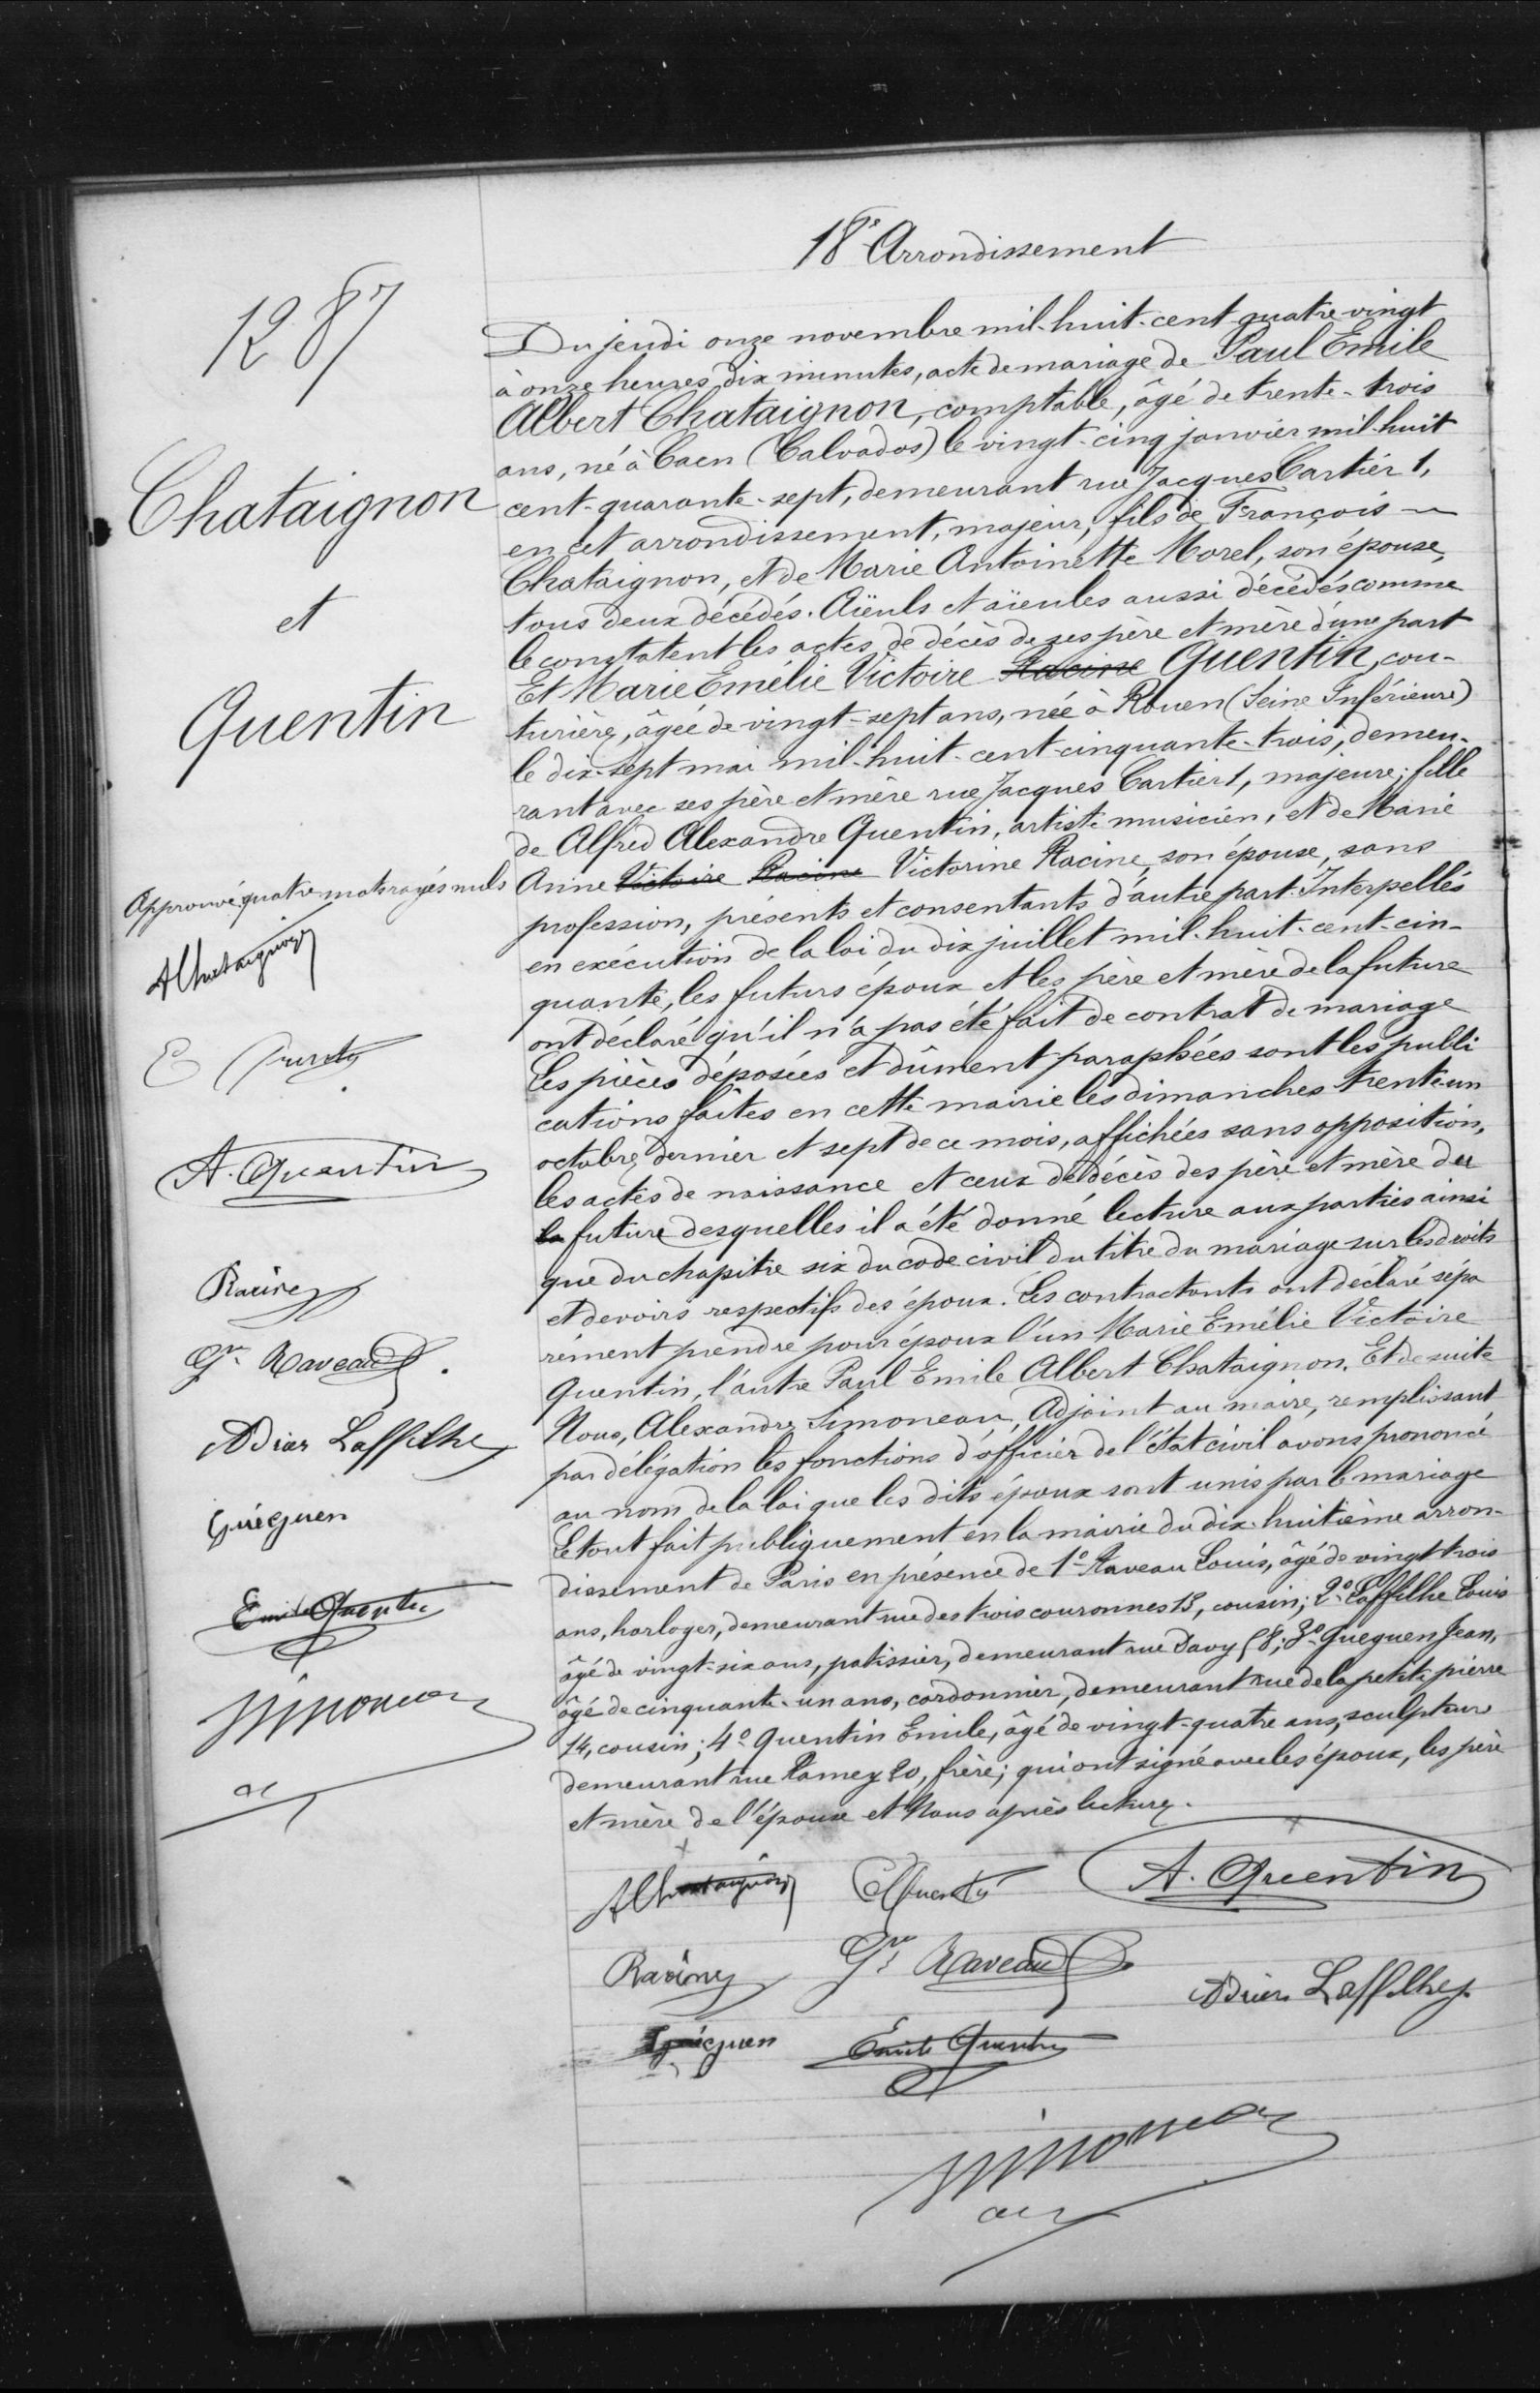1287
Chataignon
et
Quentin
18° Arrondissement
Du jeudi onze novembre mil-huit-cent-qautre vingt
à onze heures dix minutes, acte de mariage de Paul Emile
Albert Chataignon, comptable, âgé de trente-trois
ans, né à Caen (Calvados) le vingt-cinq janvier mil-huit
cent-quarante-sept, demeurant rue Jacques Cartier 1,
en cette arrondissement, majeur, fils de François -
Chataignon, et de Marie Antoinette Morel, son épouse,
tous deux décédés. Aïeuls et aïeules aussi décédés, comme
le constatent les actes de décès de ses père et mère d'une part
Et Marie Emilie Victoire Adeline Quentin, cou-
turière, âgé de de vingt-sept ans, née à Rouen (Seine Inférieure)
le dix-sept mai mil-huit-cent-cinquante-trois, demeu-
rant vec ses père et mère rue Jacques Cartier 1, majeure, fille
de Alfred Alexandre Quentin, artiste musicien, et de Marie
Anne Victoire Racine Victorine Racine, son épouse, sans
profession, présents et consentant d'autre part. Interpellés
en exécution de la loi du dix juillet mil-huit-cent-cin-
quante, les futurs époux et les père et mère de la future
ont déclaré qu'il n'a pas été fait de contrat de mariage
Les pièces déposées et dûment paraphées sont les publi
cations faites en cette mairie les dimanches trente-un
octobre dernier et sept de ce mois, affichées sans opposition,
les actes de naissance et ceux de décè des père et mère du
la future desquelles il a été donné lecture aux parties ainsi
que du chapitre six du code civildu titre du mariage sur les droits
et devoirs respectifs des époux. Les contractants ont déclaré sépa
rément prendre pour époux l'un Marie Emilie Victoire
Quentin, l'autre Paul Emile Albert Chataignon, Et de suite
Nous, Alexandre Simoneau, Adjoint au maire, remplissant
par délégation les fonctions d'officier de l'état civil avons prononcé
au nom de la loi que les dits époux sont unis par le mariage
Le tout fait publiquement en la mairie du dix-huitième arron-
dissement de Paris en présence de 1° Raveau Louis, âgé de vingt-trois
ans, horloger, demeurant rue des trois couronnes, 13, cousin; 2° Laffilhe Louis
âgé de vingt-six ans, patissier, demeurant ru Savy 58; 3° Gueguen Jean,
âgé de cinquante-un ans, cordonnier, demeurant rue de la petite pierre
14, cousin; 4° Quentin Emile, âgé de vingt-quatre ans, sculpteur
demeurant rue Lamey 20, frère; qui ont signé avec les époux, les père
et mère de l'époux et Nous après lecture.
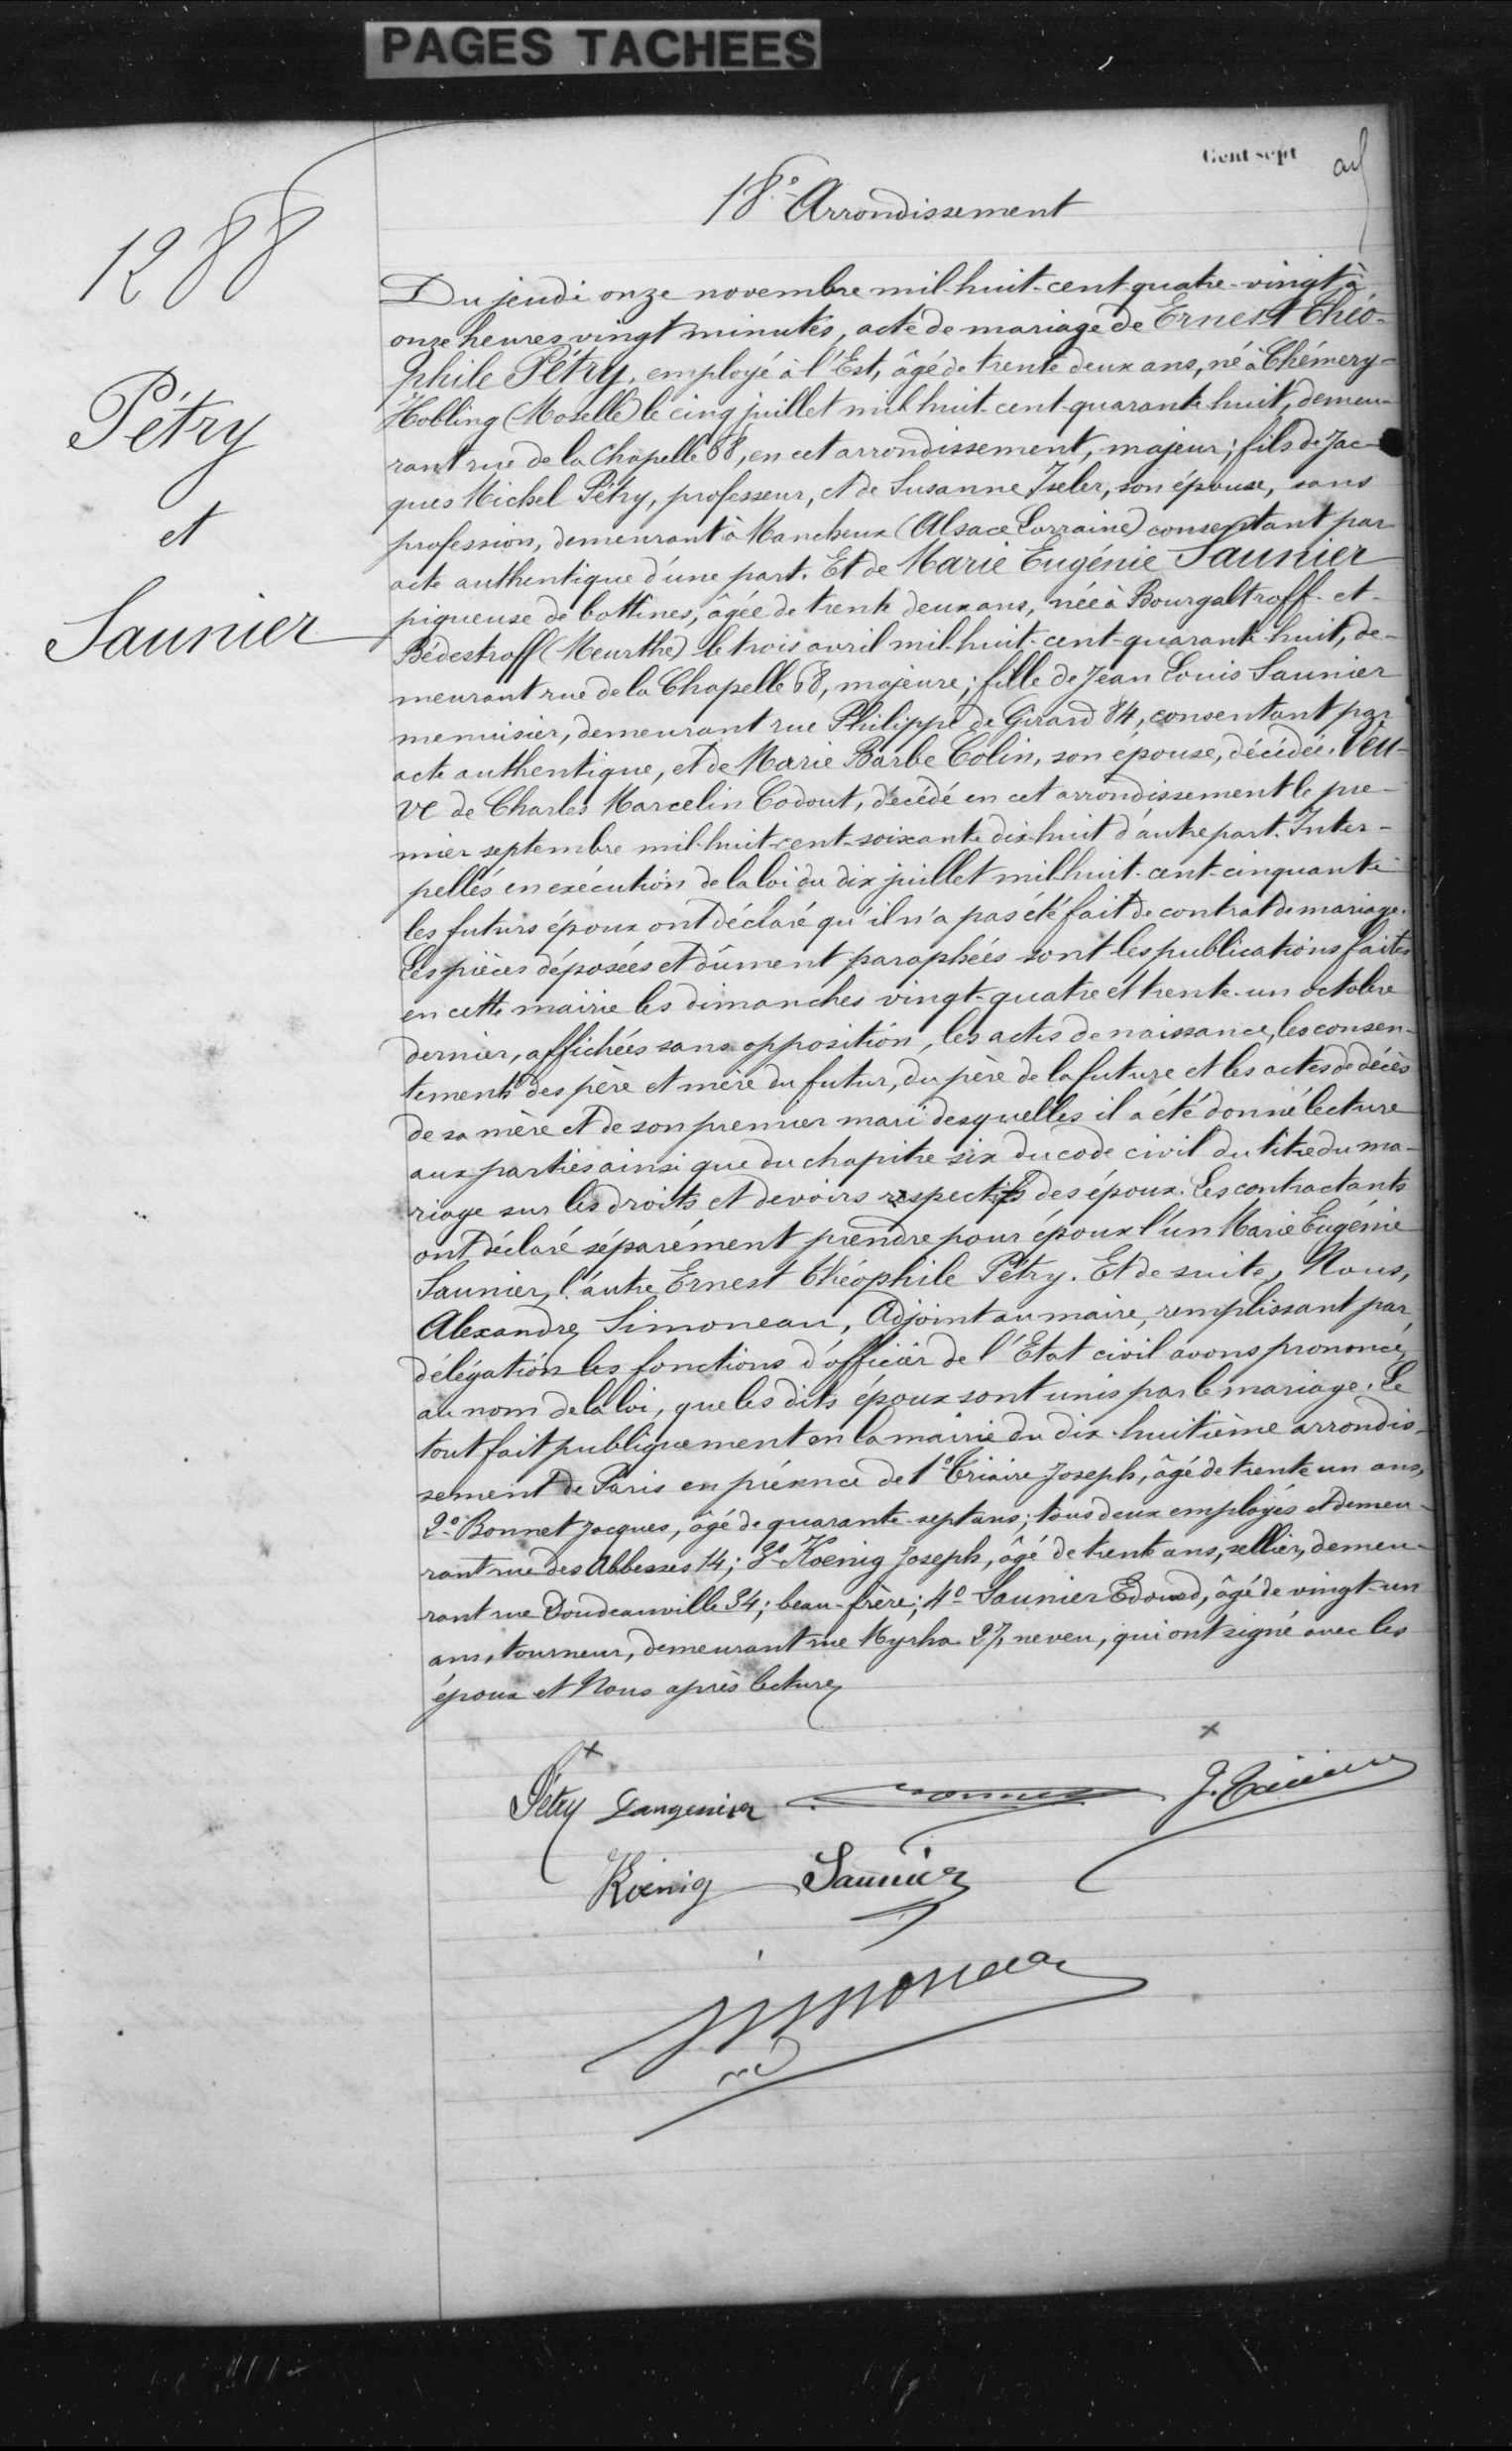1288
Pétry
et
Saunier
18° Arrondissement
Du jeudi onze novembre mil huit cent quatre-vingt à
onze heure vingt minutes, acte de mariage de Ernest Théo-
phile Pétry, employé à l'Etat, âgé de trente deux ans, né à Chénery-
Hobling (Moselle) le cinq juillet mil huit-cent-quarante huit, demeur-
rant rue de la Chapelle 58, en cet arrondissement, majeur; fils de Jac-
ques Michel Pétry, professeur, et de Suzanne Jules, son épouse, sans
profession, demeurant à Manebeux (Alsace-Lorraine) consentant par
acte authentique d'une part. Et de Marie EUgénie Saunier
piqueuse de bottines, âgée de trente deux ans, née à Bourgaltroff-et-
Bédestroff (Meurhte) le trois avril mil-huit-cent-quarante huit, de-
meurant rue de la Chapelle 68, majeure; fille de Jean Louis Saunier
menuisier, demeurant rue Philippe de Gérard 84, consentant par
acte authentique, et de Marie Barbe Colin, son épouse, décédée. Veu-
ve de Charles Marcelin Codout, décédé en cet arrondissement le pre-
mier septembre le mil-huit-cent-soixante-dix-huit d'autre part. Inter-
pellés en exécution de la loi du dix juillet mil-huicent-cinquante
les futurs époux ont déclaré qu'il n'a pas été fait de contrat de mariage.
Les pièces déposées et dûment paraphées sont les publications faites
en cette mairie les dimanches vingt-quatre et trente-un octobre
dernier, affichées sans opposition, les actes de naissance, les consen-
tements des père et mère du future, du père de la future et les actes de décès
de sa mère et de son premier marie desquelles, il a été donné lecture
aux parties ainsi que du chapitre six du code civil du titre du ma-
riage sur les droits et devoirs respectifs des époux. Les contractants
ont déclaré séparément prendre pour époux l'un Marie Eugénie
Saunier, l'autre Ernest Théophile Petry. Et de suite, Nous
Alexandre Simoneau, Adjoint au maire, remplissant par
délégation les fonction d'officier de l'Etat civil avons prononcé
au nom de la loi, que les dits époux sont unis par le mariage. Le
tout fait publiquement en la mairie du dix-huitième arrondis-
sement de Paris en présence de 1° Triaire Joseph, âgé de trente un ans,
2° Bonnet Jacques, âgé de quarante-sept ans; tous deux employés et demeur-
rant rue des Abbesses 14; 3° Koenig Joseph, âgé de trente ans, sellier, demeu-
rant rue Doudeauville 34; beau-père; 4° Saunier Edouard, âgé de vingt-un
ans, tourneur, demeurant rue Myrpha 27, neveu, qui ont signé avec les
époux et Nous après lecture.